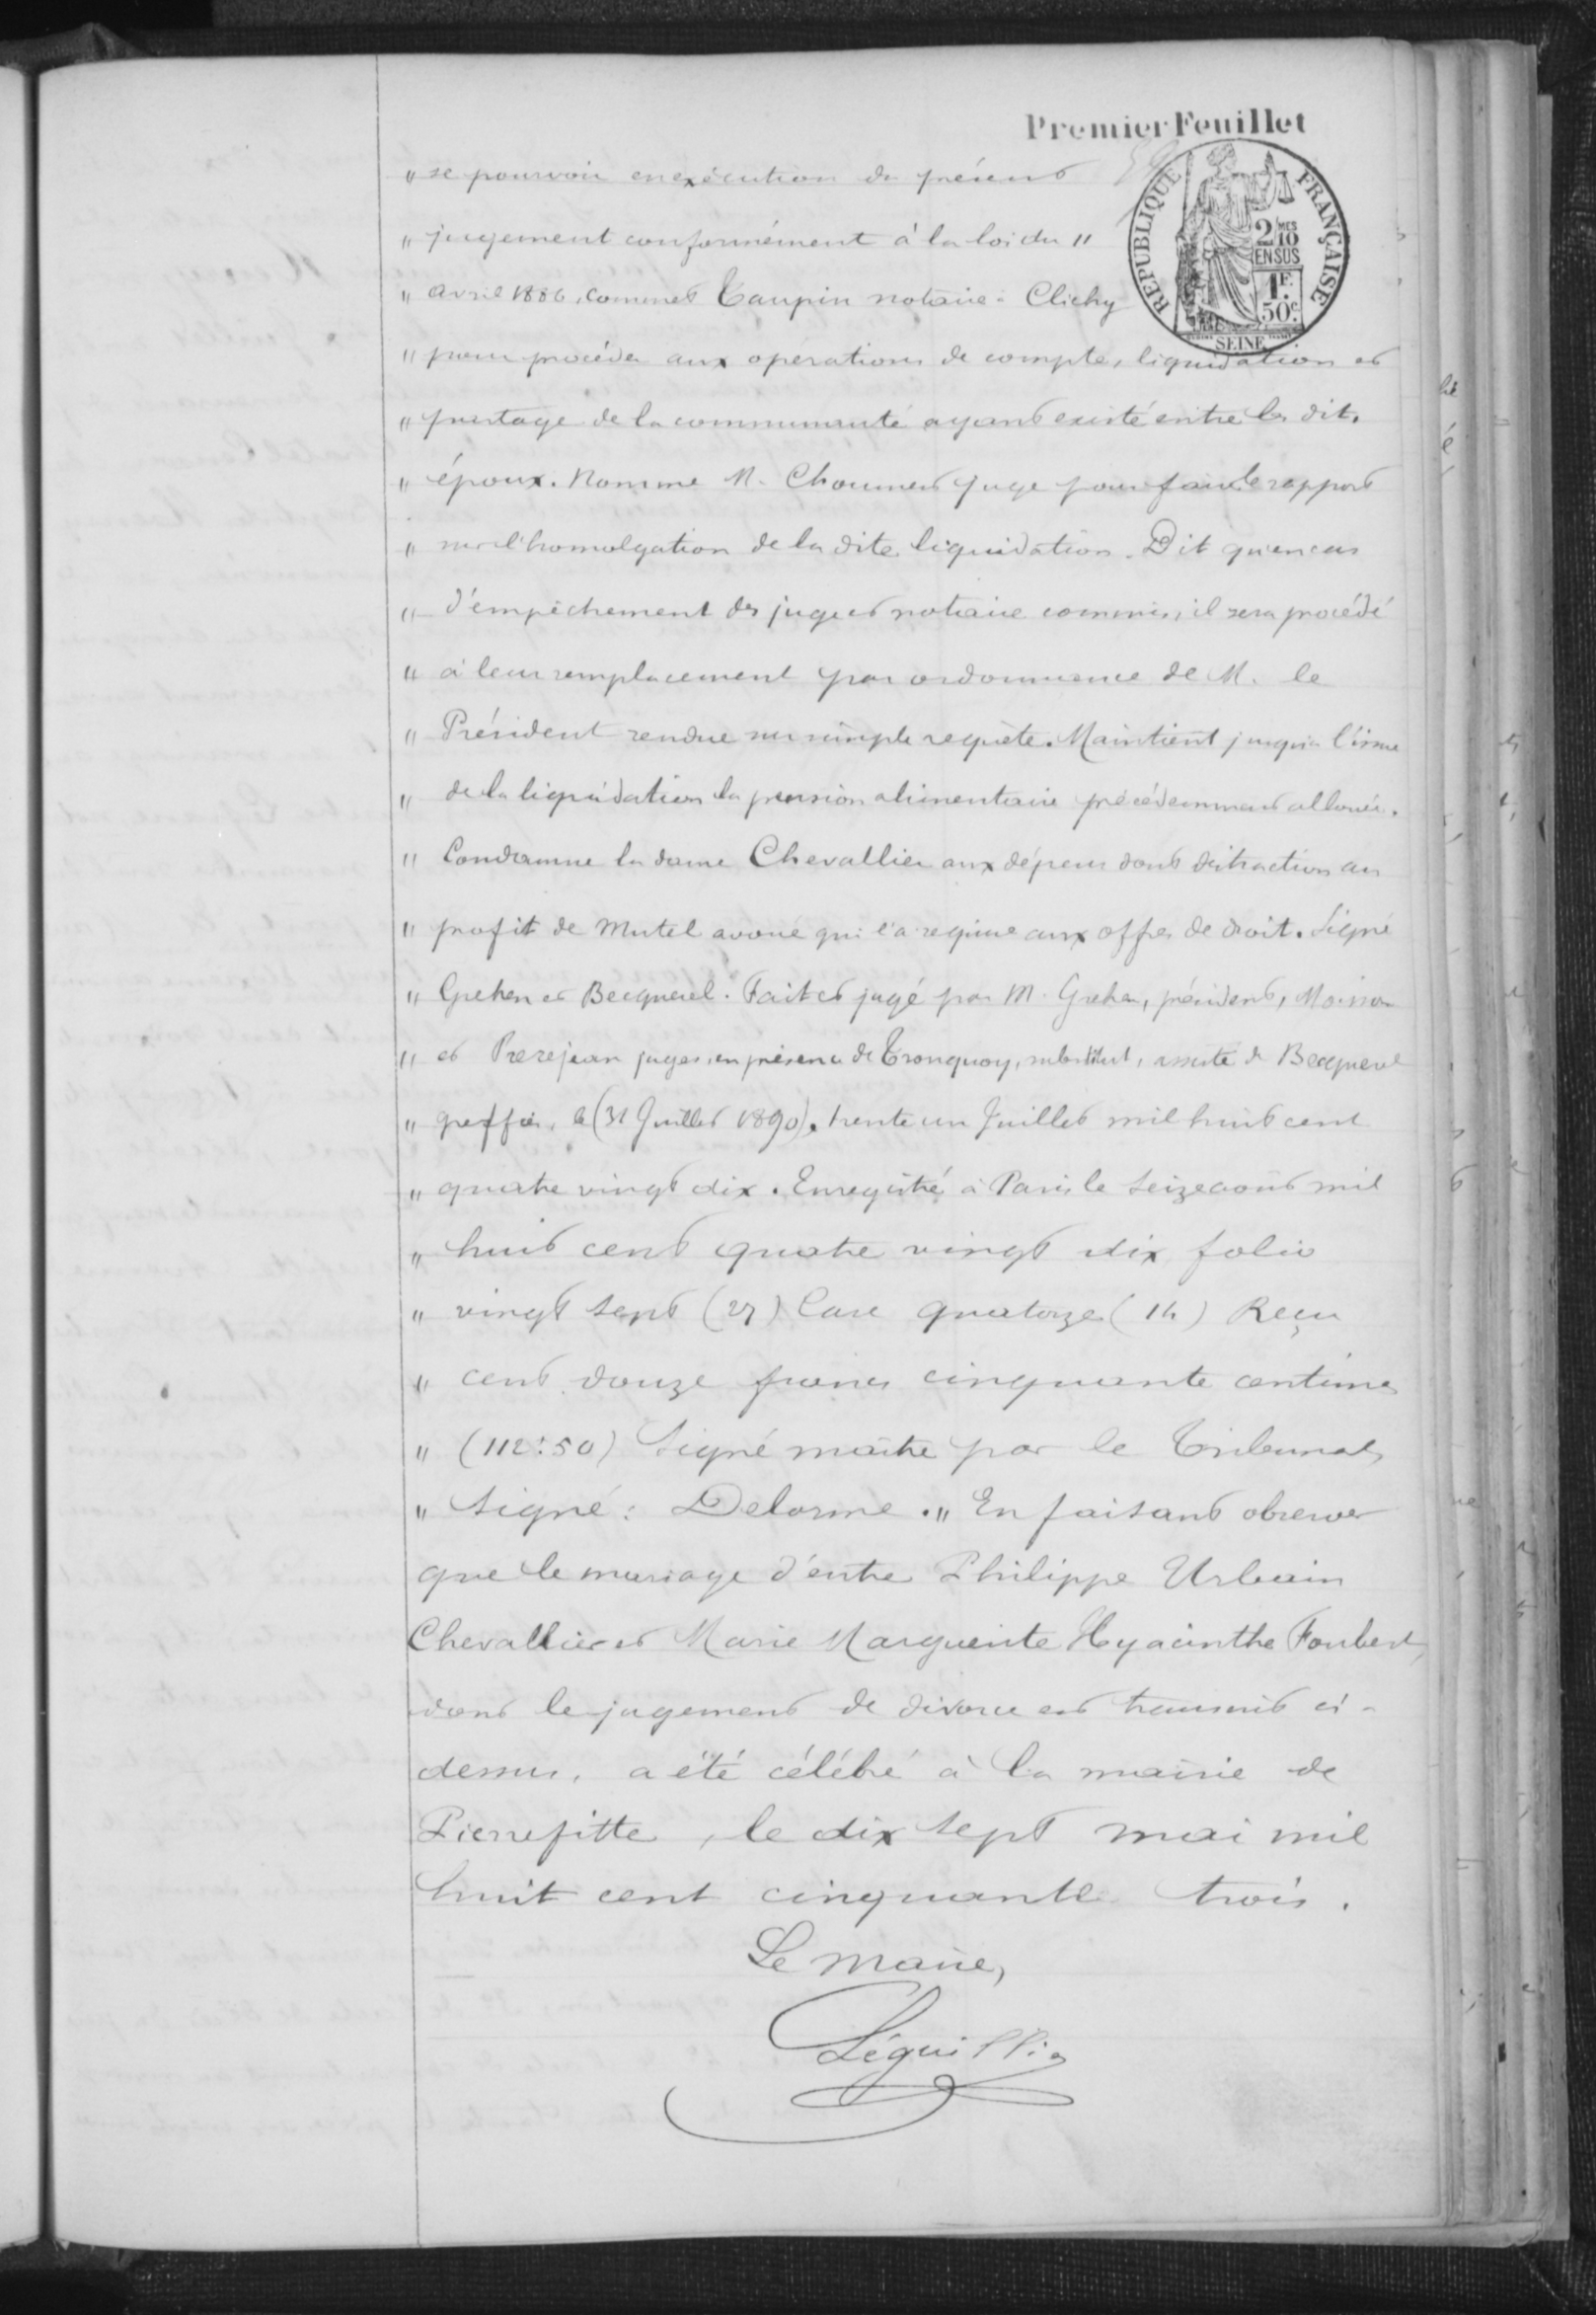se pourvoir en exécution du présent
jugement conformément à la loi du 11
avril 1886, comme Laupin notaire-Clichy
pour procéder aux opérations de compte, liquidation et
partage de la communauté ayant existé entre les dits
époux. Nomme 11, Choumert juge pour faire le rapport
sur l'homolgation de la dite liquidation. Dit qu'en cas
d'empêchement des juges et notaire comme il sera procédé
à leur remplacement par ordonnance de 11. le
Président rendu sur simple requête. Maintient jusqu'à l'issue
de la liquidation la pension alimentaire précédemment allouée
Condamne la dame Chevallier aux dépens dont distraction au
profit de Mutel avoue qui l'a requis aux offre de droit. Ligné
Greten et Becquerel. Fait et jugé par M Guste, président, Moissier
Et Prerejean juges, en présence de Tronquay, assiste de Becquerel
Greffoi, le (31 Juillet 1890) trente un Juillet mil huit cent
quatre vingt dix: Enregistré à Paris le seize août mil
huit cent quatre vingt dix falu
vingt sept (27) Care quatorze (14) Reçu
cent douze francs cinquante centimes
(112: 50) signé maître par le Tribunal
signé: Delorme. En faisant observe
que le mariage d'entre Philippe urbain
Chevallier Marie Marguerite Hyacinthe Foubert
dont le jugement de divorce est terminé ci-
dessus, a été célébré à la mairie de
Pierrfitte, le dix sept mai mil
huit cent cinquante trois.
Le maire,